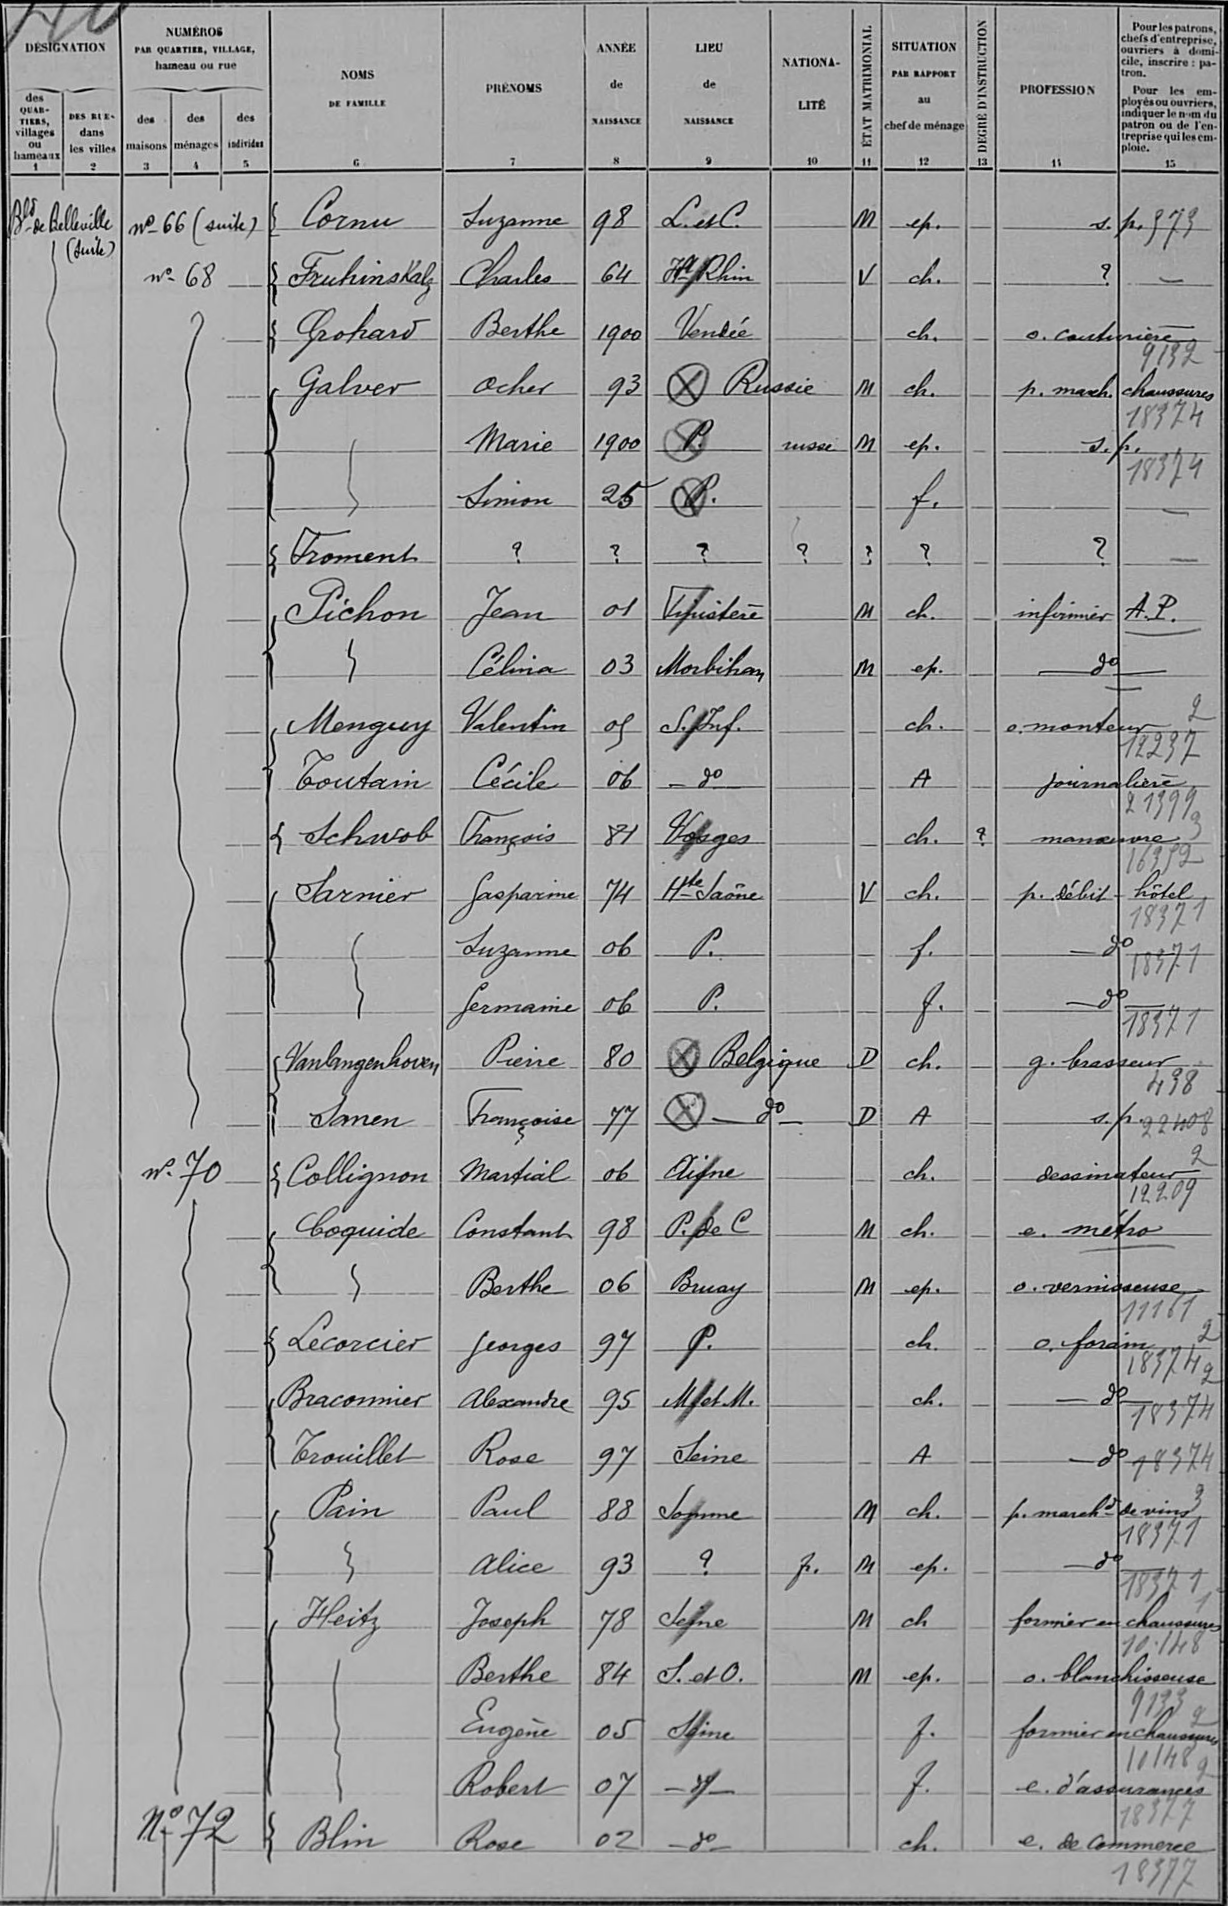Cornu/Suzanne/98/L et C /¤/M/ep /¤/s p /573
Fruhinskalz/Charles/64/Ht Rhin/¤/V/ch /¤/¤/¤
Grohard/Berthe/1900/Vendée/¤/¤/ch /¤/o couturière/¤
Galver/Ocher/93/Russie/¤/M/ch /¤/p march chaussures/¤
¤/Marie/1900/P /russe/M/ep /¤/s p /¤
¤/Simon/25/P /¤/¤/f /¤/¤/¤
Froment/¤/¤/¤/¤/¤/¤/¤/¤/¤
Pichon/Jean/01/Finistère/¤/M/ch /¤/infirmier/A P
¤/Célina/03:Morbihan/¤/M/ep /¤/d°/¤
Menguy/Valentin/05/S Inf /¤/¤/ch /¤/o monteur/¤
Toutain/Cécile/06/d°/¤/¤/A/¤/jounalière/¤
Schwob/François/81/Vosges/¤/¤/ch /¤/manoeuvre/¤
Sarnier/Gasparine/74/Hte Saône/¤/V/ch /¤/p début-hôtel/¤
¤/Suzanne/06/P /¤/¤/f /¤/d°/¤
¤/Germaine/06/P /¤/¤/f /¤/d°/¤
Vanlangenhoven/Pierre/80/Belgique/¤/D/ch /¤/g brasseur/¤
Sanen/Françoise/77/d°/¤/D/A/¤/s p /22408
Collignon/Martial/06/Aisne/¤/¤/ch /¤/dessinateur/¤
Coquide/Constant/98/P de C/¤/M/ch /¤/e métro/¤
¤/Berthe/06/Bruay/¤/M/ep /¤/o vernisseuse/¤
Lecorcier/Georges/97/P /¤/¤/ch /¤/o forian/¤
Braconnier/Alexandre/95/M et M /¤/¤/ch /¤/d°/¤
Trouillet/Rose/97/Seine/¤/¤/A/¤/d°/18374
Pain/PAul/88/Somme/¤/M/ch /¤/p marchd de vins/¤
¤/Alice/93/¤/f /M/ep /¤/d°/¤
Heitz/¤/Joseph/78/Seine/¤/M/ch/¤/formier en chaussures/¤
¤/Berthe/84/S et O /¤/M/ep /¤/o blanchisseuse/¤
¤/Eugène/05/Seine/¤/¤/f /¤/formier en chaussures/¤
¤/Robert/07/d°/¤/¤/f /¤/e d'assurances/¤
Blin/Rose/02/d°/¤/¤/ch /¤/e de commerce/¤
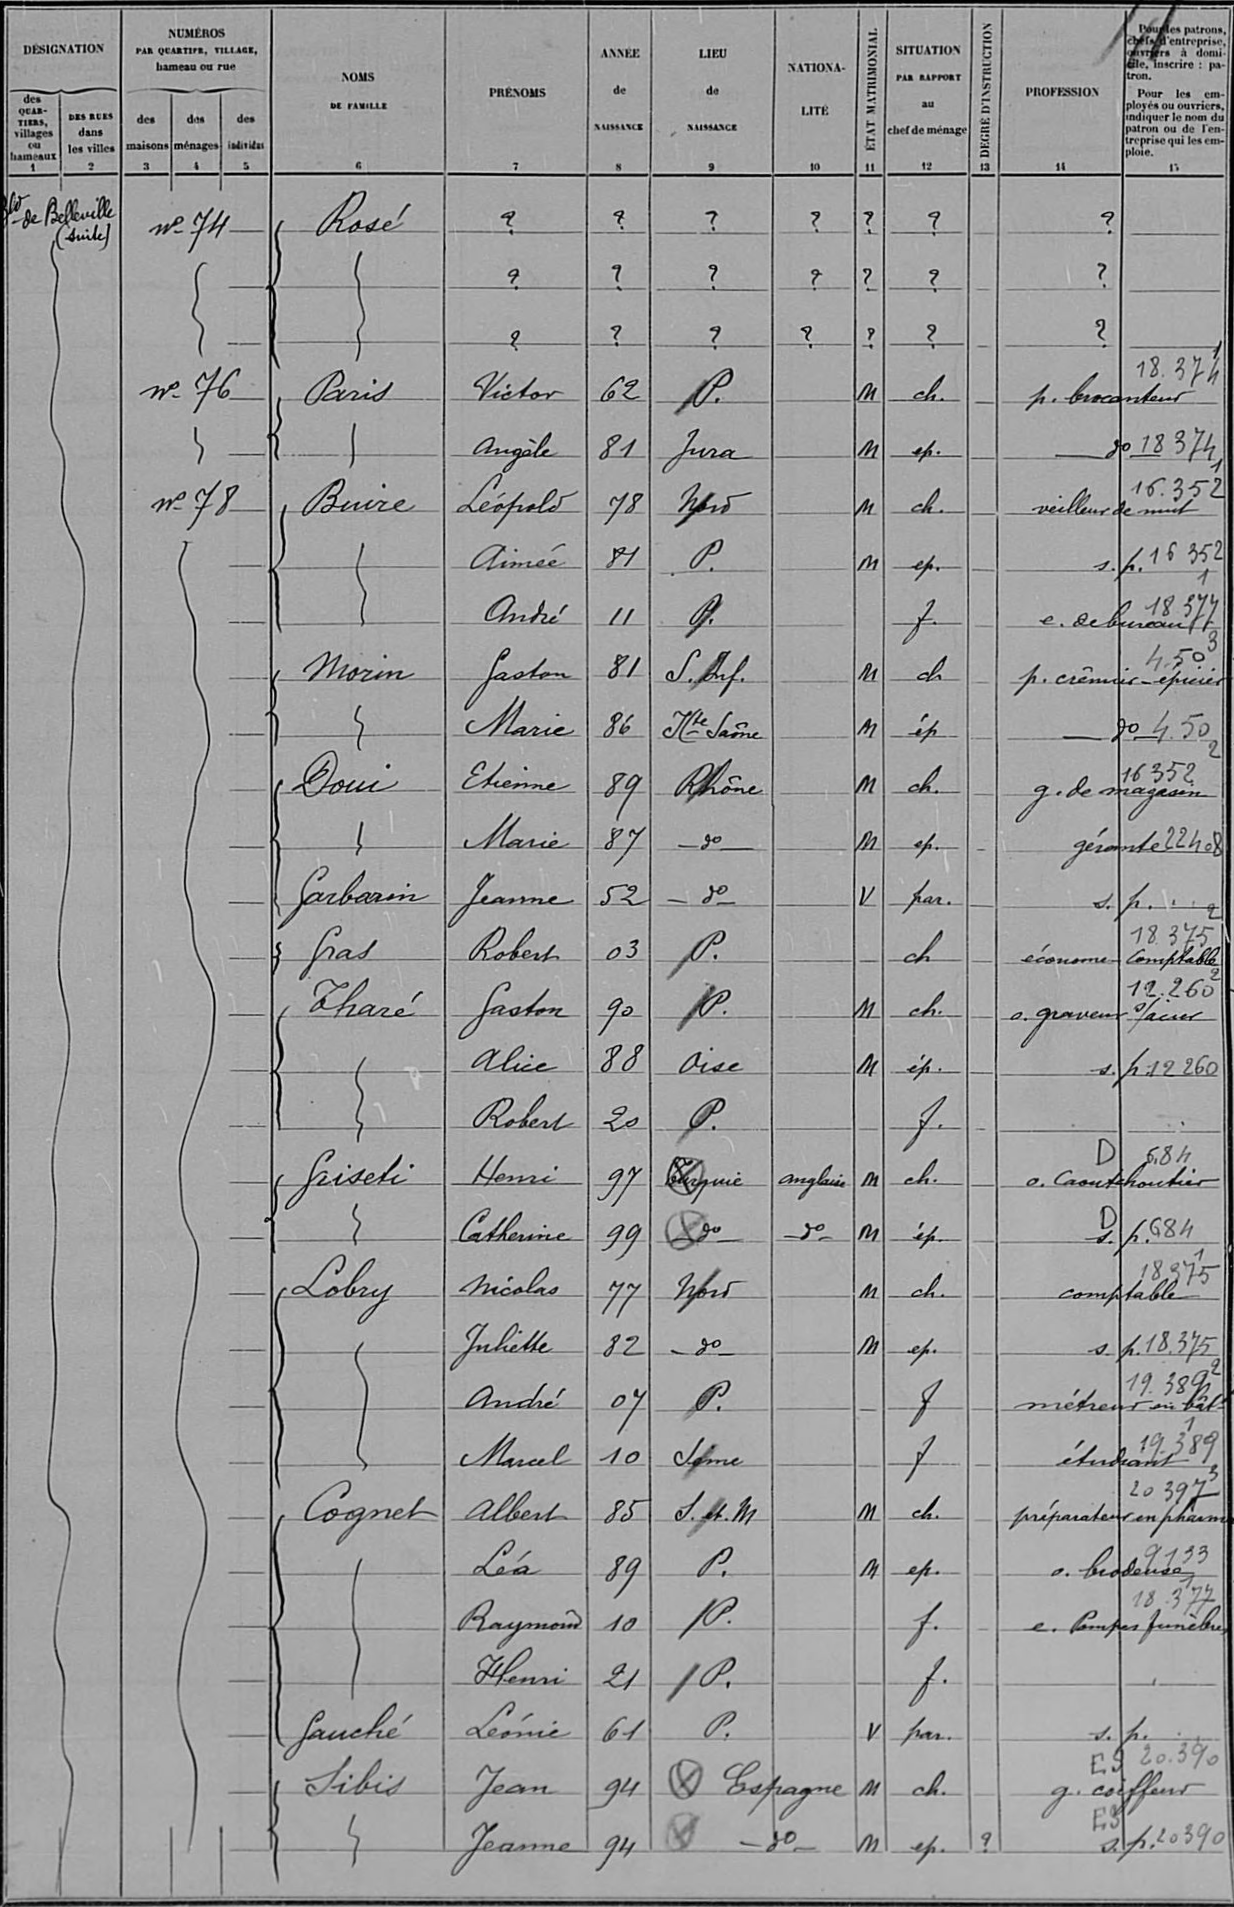Rosé/¤/¤/¤/¤/¤/¤/¤/¤/¤
¤/¤/¤/¤/¤/¤/¤/¤/¤/¤
¤/¤/¤/¤/¤/¤/¤/¤/¤/¤
Paris/Victor/62/P /¤/M/ch /¤/p brocanteur/¤
¤/Angèle/81/Jura/¤/M/ep /¤/d°/18374
Buire/Léopold/78/Nord/¤/M/ch /¤/veilleur de nuit/¤
¤/Aimée/81/P /¤/m/ep /¤/s p /16352
¤/André/11/P /¤/¤/f /¤/e de bureau/¤
Morin/Gaston/81/S Inf/¤/M/ch/¤/p crêmier-épicier/¤
¤/Marie/86/Hte Saône/¤/M/ép/¤/d°/450
Doui/Etienne/89/Rhône/¤/M/ch /¤/g de magasin/¤
¤/MArie/87/d°/¤/M/ep /¤/gérante/22408
Garbarin/Jeanne/52/d°/¤/V/par /¤/s p
Gras/Robert/03/P /¤/¤/ch/¤/économe comptable/¤
Tharé/Gaston/90/P /¤/M/ch /¤/o graveur s acier/¤
¤/Alice/88/Oise/¤/M/ép /¤/s p /12260
¤/Robert/20/P /¤/¤/f /¤/¤/¤
Griseti/Henri/97/Turquie/anglaise/M/ch /¤/o caoutchoutier/¤
¤/Catherine/99/d°/d°/M/ép/¤/¤/s p /¤
Lobry/Nicolas/77/Nord/¤/M/ch /comptable/¤
¤/Juliette/82/d°/¤/M/ep /¤/s p /18375
¤/André/07/P /¤/¤/f/¤/métreur en bât/¤
¤/MArcel/10/Seine/¤/¤/f/¤/étudiant/¤
Cognet/Albert/85/S et M/¤/M/ch /¤/préparateur en pharma/¤
¤/Léa/89/P /¤/M/ep /¤/o brodeuse/¤
¤/Raymond/10/P /¤/¤/f /¤/e Pompes funèbres/¤
¤/Henri/21/P /¤/¤/f /¤/¤/¤
Gauché/Léonie/61/P /¤/V/par /¤/s p /¤
Sibis/Jean/94/Espagne/M/ch /¤/g coiffeur/¤
¤/Jeanne/94/d°/¤/M/ep /¤/s p /20390
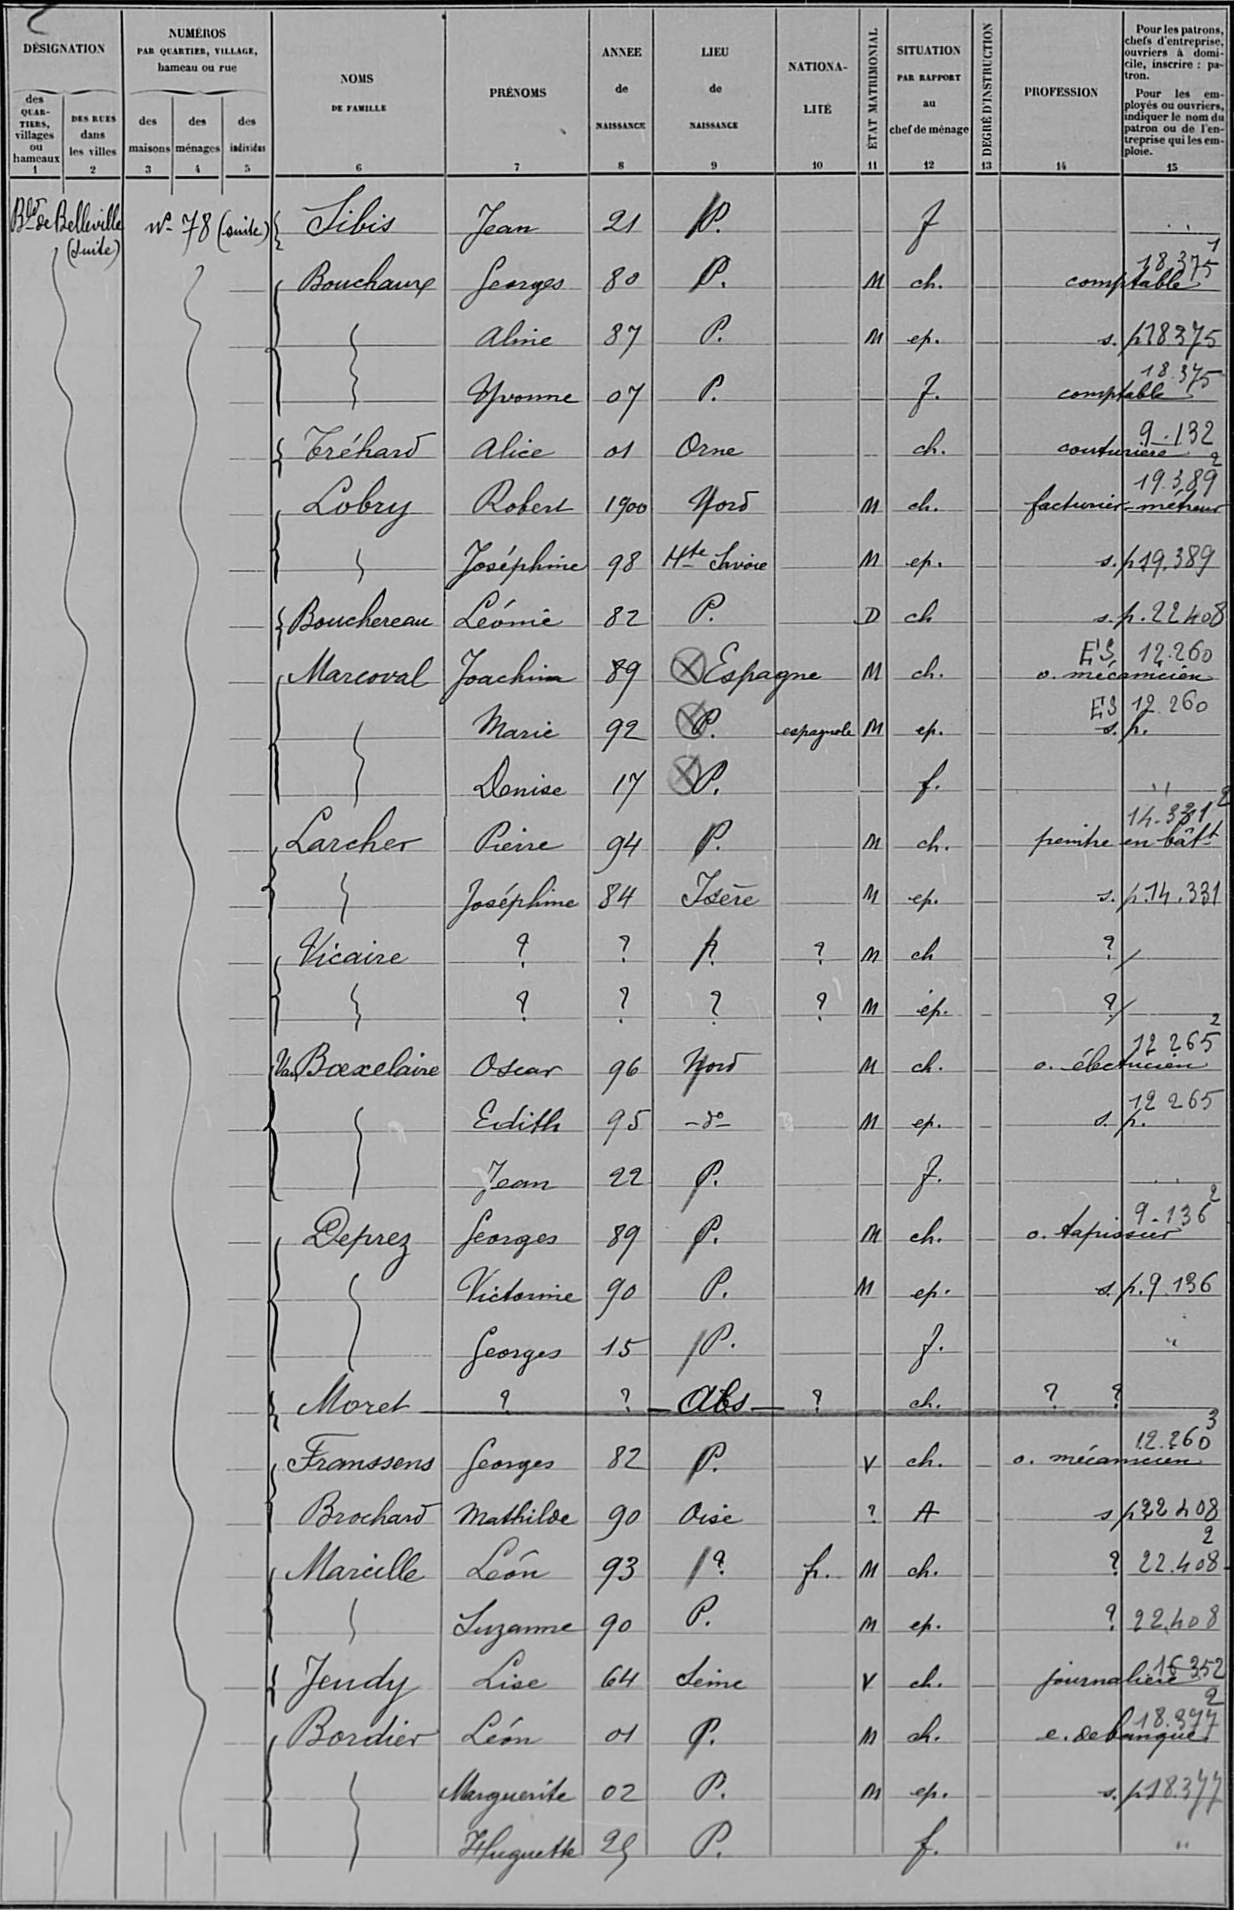Sibis/Jean/21/P /¤/¤/f/¤/¤/¤
Bouchaux/Georges/80/P /¤/M/ch /¤/comptable/¤
¤/Aline/87/P /¤/M/ep /¤/s p /18375
¤/Yvonne/07/P /¤/¤/f /¤/comptable/¤
Tréhard/Alice/01/Orne/¤/¤/ch /¤/couturière/¤
Lovry/Robert/1900/Nord/¤/M/ch /¤/facturier-métreur/¤
¤/Joséphine/98/Hte Savoie/¤/M/ep /¤/s p /19389
Bouchereau/Léonie/82/P /¤/D/ch/¤/s p /22408
Marcoval/Joachim/89/Espagne/¤/M/ch /¤/o mécanicien/¤
¤/Marie/92/P /espagnole/M/ep /¤/s p /¤
¤/Denise/17/P /¤/¤/f /¤/¤/¤
Larcher/Pierre/94/P /¤/M/ch /¤/peintre en bâtt/¤
¤/Joséphine/84/Isère/¤/M/ep /¤/s p /14331
Vicaire/¤/¤/¤/¤/M/ch/¤/¤/¤
¤/¤/¤/¤/M/ép /¤/¤/¤
Van Boexelaire/Oscar/96/Nord/¤/M/ch /¤/o électricien/¤
¤/edith/95/d°/¤/M/ep /s p /12265
¤/Jean/22/P /¤/¤/f /¤/¤/¤
Deprez/Georges/89/P /¤/M/ch /¤/o tapissier/¤
¤/Victorine/90/P /¤/M/ep /¤/s p /9136
¤/Georges/15/P /¤/¤/f /¤/¤/¤
Moret/¤/¤/-Abs-/¤/¤/ch /¤/¤/¤
Franssens/Georges/82/P /¤/v/ch /¤/o mécanicien/¤
Brochard/MAthilde/90/Oise/¤/¤/A/¤/sp/22408
Marcille/Léon/93/¤/fr /M/ch /¤/¤/22408
¤/Suzanne/90/P /¤/M/ep /¤/¤/22408
Jendy/Lise/64/Seine/¤/V/Ch /¤/journalière/¤
Bordier/Léon/01/P /¤/M/ch /¤/e de banque/¤
¤/MArguerite/02/P /¤/M/ep /¤/s p /18377
¤/Huguette/25/P /¤/¤/f /¤/¤/¤